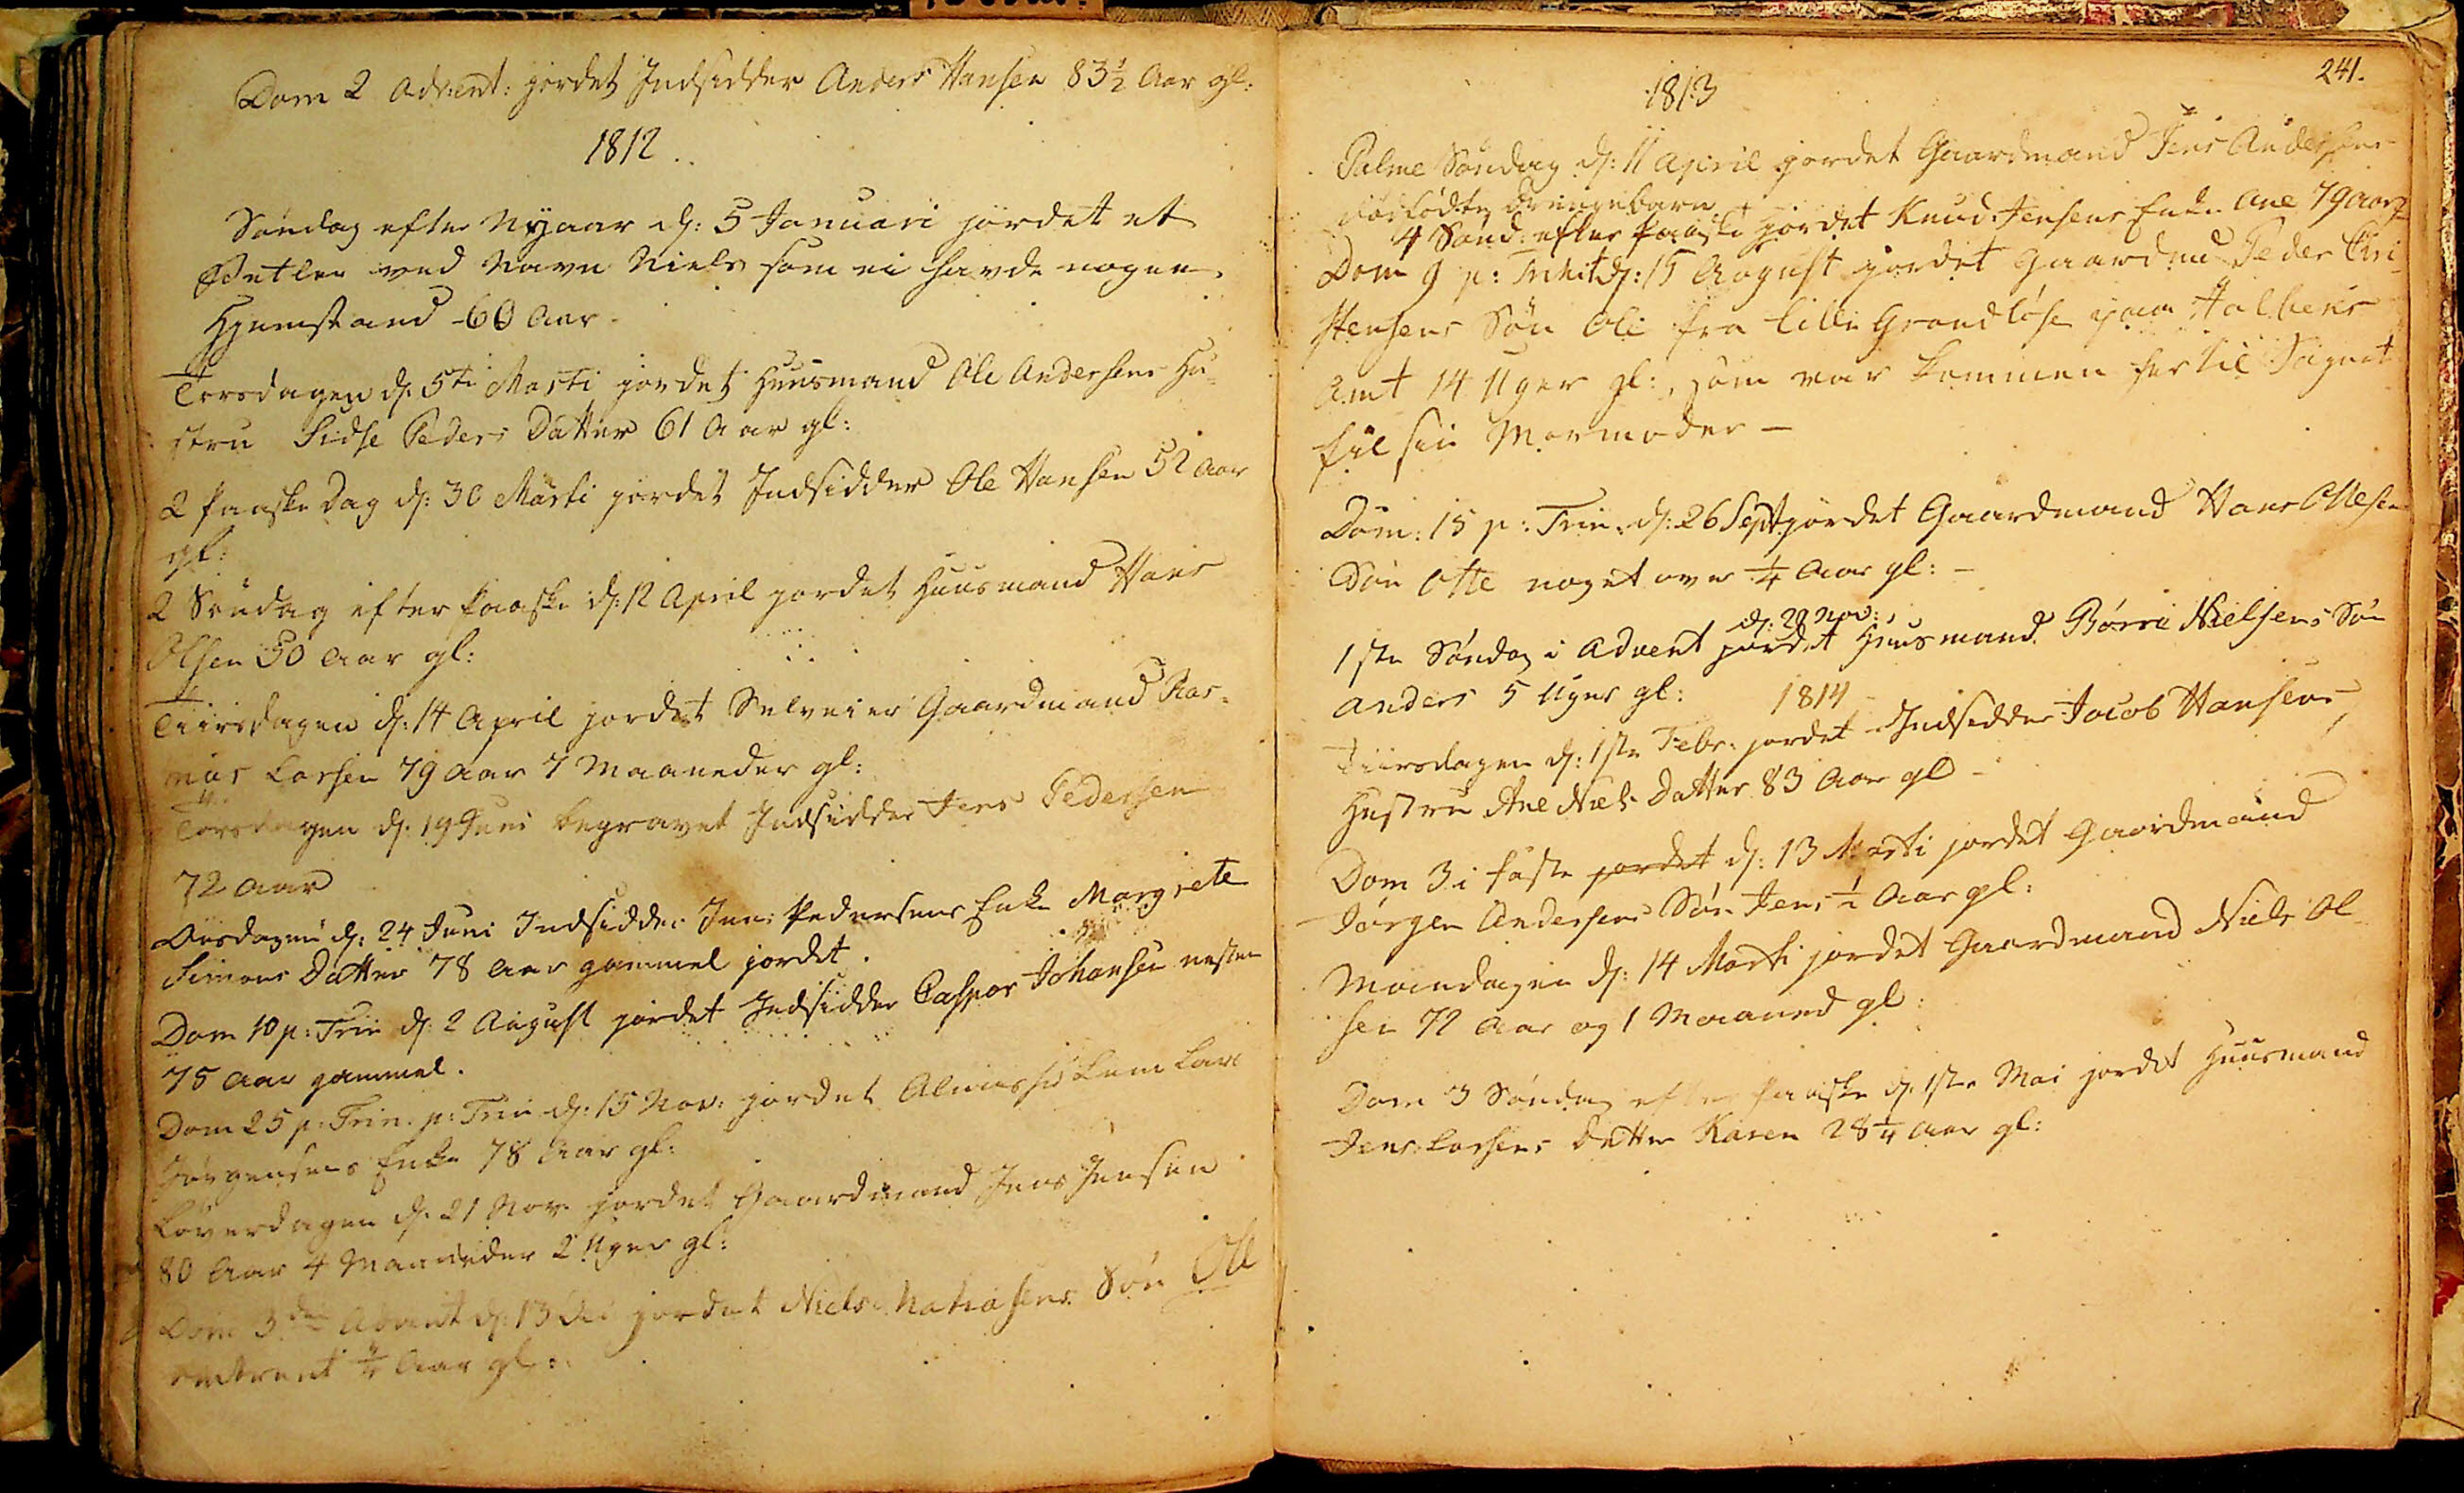Dom 2 Advent: jordet Indsidder Anders Hansen 83½ Aar gl:
1812
Søndag efter Nyaar d: 5 Januari jordet et
Betler## ved Navn Niels som ei havde nogen
Hjemstaed - 60 Aar
Torsdagen d: 5te Marti jordet Huusmand Ole Andersens Hu¬
strue Sidse Peders Datter 61 Aar gl:
2 Paaske Dag d: 30 Marti jordet Indsidder Ole Hansen 52 Aar
gl:
2 Søndag efter Paaske d: 12 April jordet Huusmand Hans
Olsen 50 Aar gl:
Tiirsdagen d: 14 April jordet Selveier Gaardmand Ras¬
mus Larsen 79 Aar 7 Maaneder gl:
Torsdagen d: 19 Juni begravet Indsidder Jens Pedersen
72 Aar
Onsdagen d: 24 Juni Indsidder Jens Pedersens Enke Margrete
Simons Datter 78 Aar gammel jordet.
Dom 10 p: Trin d: 2 August jordet Indsidder Caspor Johansen næsten
75 Aar gammel.
Dom 25 p: Trin: p: Trin d: 15 Nov: jordet Almisse Lem Lars
Jørgensens enke 78 Aar gl:
Løverdagen d:21 Nov jordet Gaardmand Jens Jensen
80 Aar 4 Maaneder 2 Uger gl:
Dom 3die Advent d: 13 Dec: jordet Niels Matiasens Søn Ole
omtrent 3/4 Aar gl:
241.
1813
Palme Søndag d: 11 April jordet Gaardmand Jens Andersens
dødfødte Drengebarn
4 Sønd: efter Paaske jordet Knud Jensens Enke Ane 79 Aar gl
Dom 9 p: Trinit d: 15 August jordet Gaardmd Peder Chri¬
stensens Søn Ole fra lille Granløse paa Holbeks
Amt 14 Uger gl:, som var kommen hertil Sognet
til sin Mormoder
Dom: 15 p: Trin: d: 26 Sept jordet Gaardmand Hans Ottesens
Søn Otte noget over 1/4 Aar gl:
d: 29 Nov:
1ste Søndag i Advent jordet Huusmand Børre Nielsens Søn 
Anders 5 Uger gl:
1814
Tiirsdagen d: 1ste Febr: jordet Indsidder Jacob Hansens
Hustrue Ane Niels Datter 83 Aar gl.
Dom 3 i Faste jordet d: 13 Marti jordet Gaardmand
Jørgen Andersens Søn Jens ½ Aar gl:
Mandagen d: 14 Marti jordet Gaardmand Niels Ol
sen 72 Aar og 1 Maaned gl:
Dom 3 Søndag efter Paaske d: 1ste Mai jordet Huusmand
Jens Larsens Datter Karen 28 1/4 Aar gl: